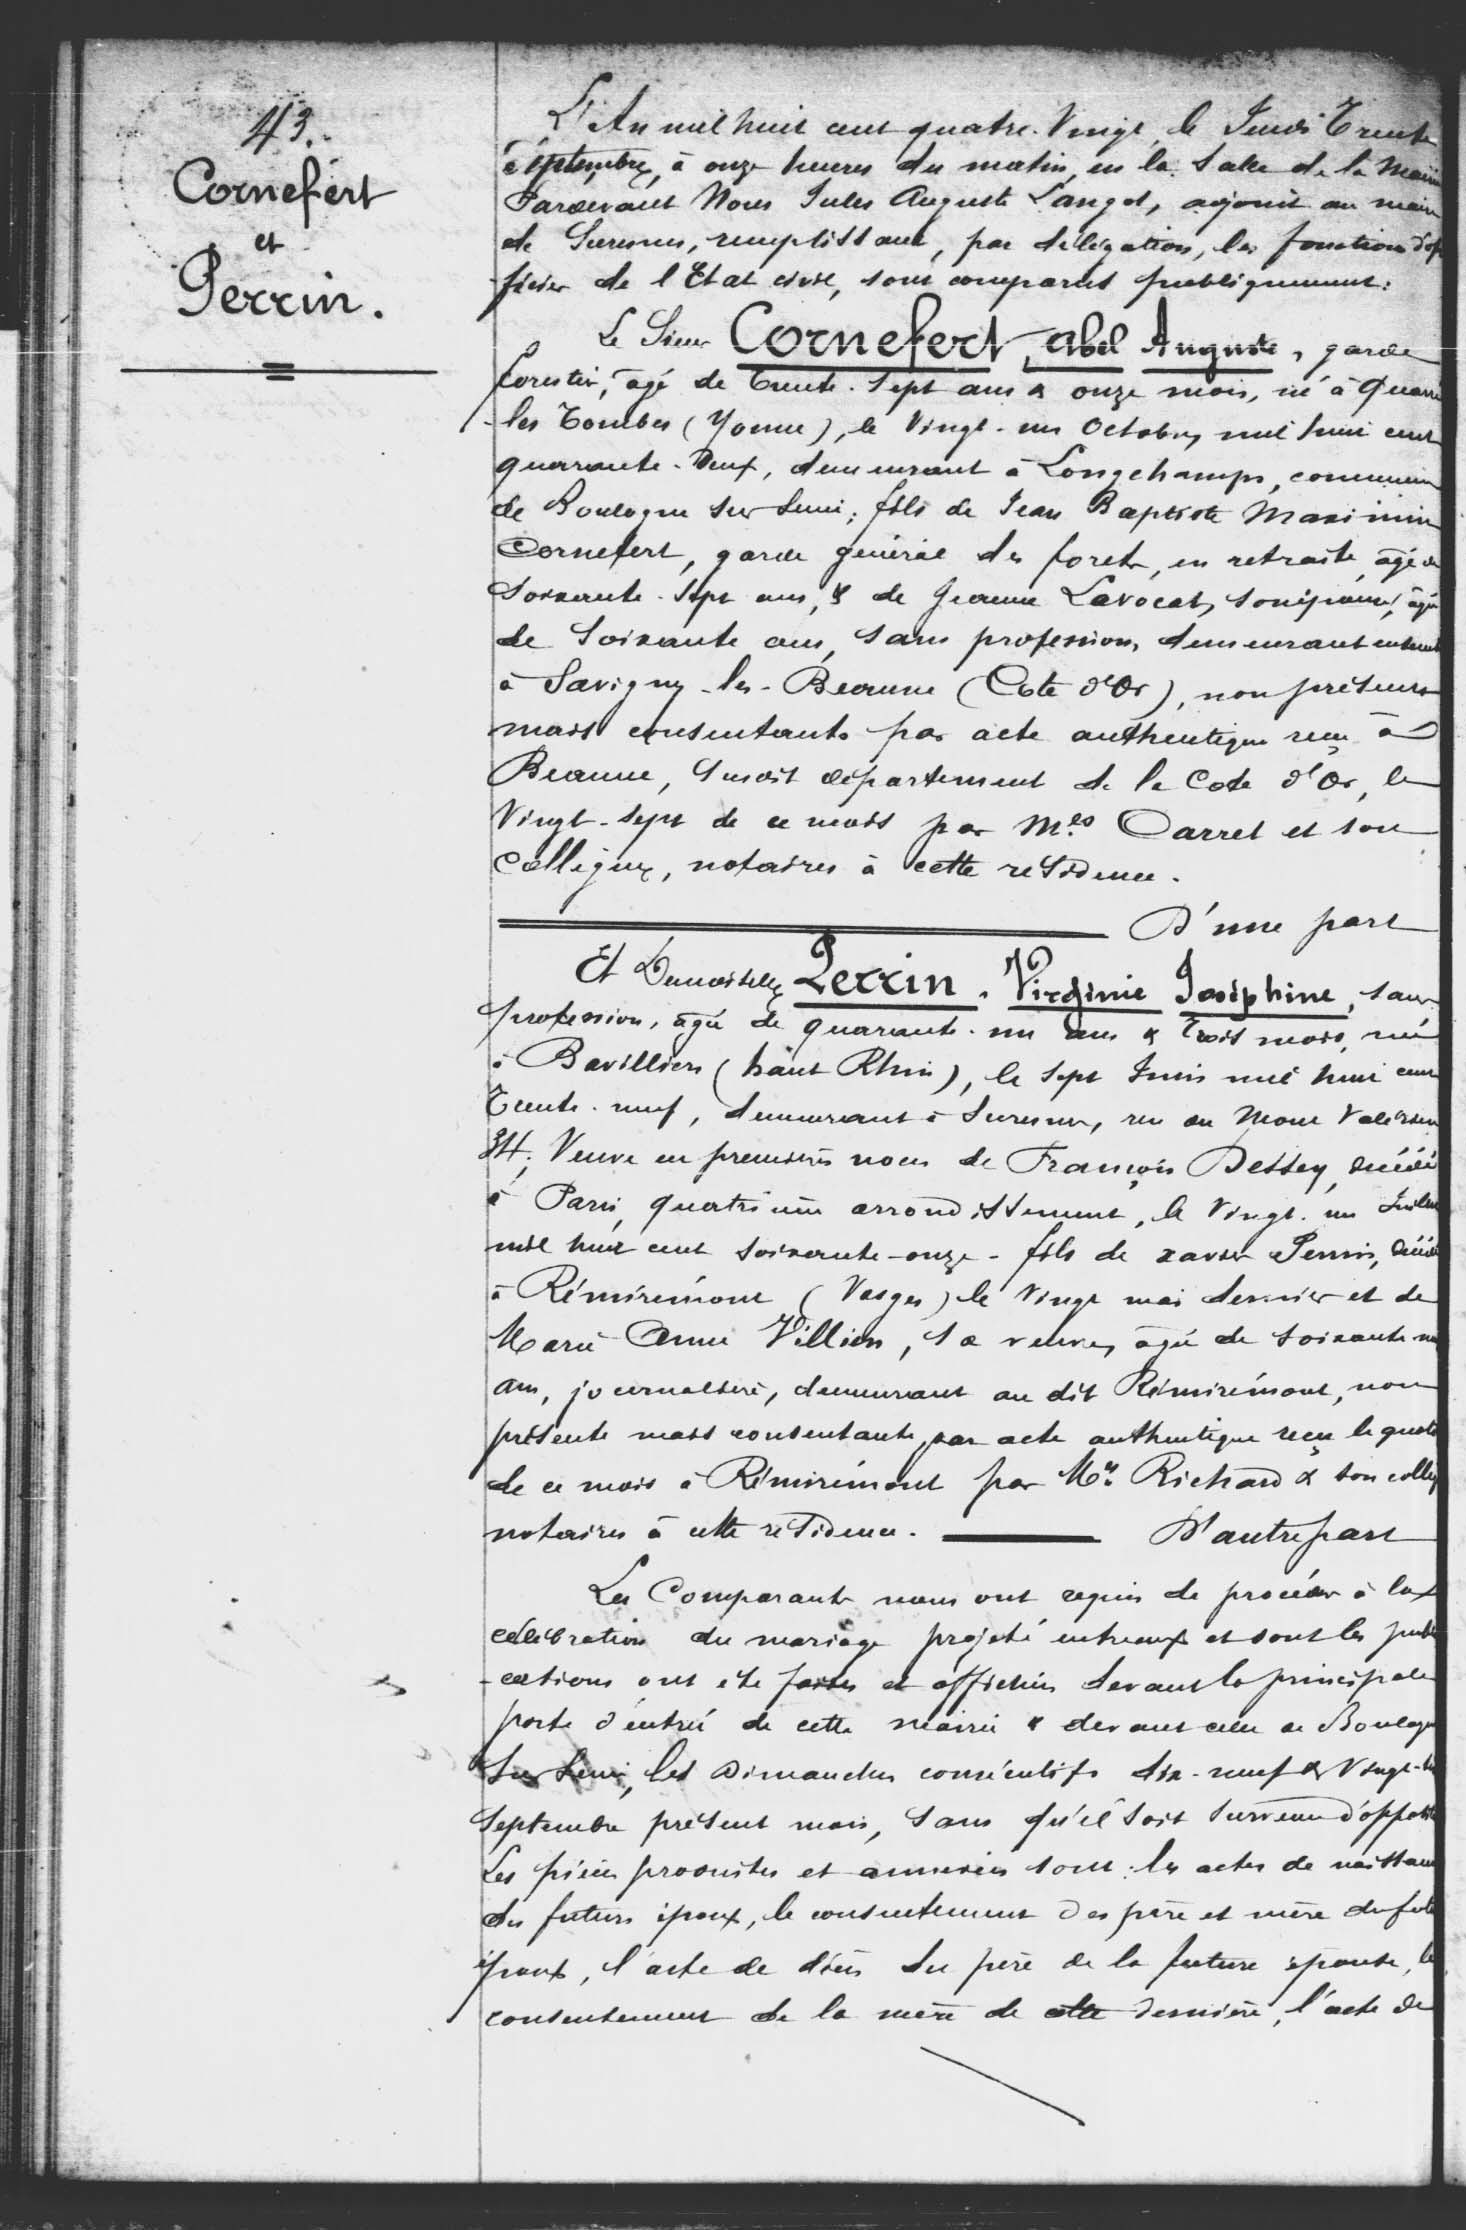43
Cornefert
et
Perrin.
L'An mil huit cent quatre Vingt le Jeudi Trente
septembre, à onze heures du matin, en la Salle de la Mairie
Pardevant Nous Jules Auguste Langot, adjoint au maire
de Turesnes, remplissant, par délégation, la fonctions d'of
ficier de l'Etat civil, sont comparus publiquement:
Le Sieur Cornefert, Abel Auguste, garde
forestier, agé de Trente sept ans & onze mois, née à Quani
les Tourbes (Yonne), le Vingt-un Octobre, mil huit cent
quarante-deux, demeurant à Longchamps, commune
de Boulogne sur Seine; fils de Jean Baptiste Maximin
Cornefert, garde général des forets, en retraite agé de
soixante-sept ans, de Jeanne Lavocat, son épouse, âgé
de Soixante ans, sans profession, demeurant ensemble
à Savigny-les-Beaunes (Cote-d'Or), non présents
mais consentants par acte authentique reçu à
Beaune, susdit département de la Cote d'Or, le
Vingt-sept de ce mois par Mes Carret et son
Collègue, notaires à cette résidence.
-D'une part
Et Damoiselle Perrin Virginie Joséphine, sans
profession, agé de quarante un ans & trois mois née
à Bavilliers (haut Rhin), le sept Juin mil huit cent
Trente-neuf, demeurant à Suresnes, rue du Mont Valérien
34; Veuve en premières noces de François Dessey, décédé
à Paris, quatrième arrondissement, le vingt-un Juillet
mil neuf cent soixante-onze-fils de Xavier Perrin, décédé
à Rémiremont (Vosges) le Vingt mai dernier et de
Marie Anne Villion, sa veuve, agé de soixante un
ans, journalière, demeurant au dit Rémiremont, non
présents mais consentants par acte authentique reçu le quatre
de ce mois à Rémiremont par Me Richard & son colleg
notaires à cette résidence. -D'autre part
Les Comparant nous ont requis de procéder à la
célébration du mariage projeté entre eux et dont les publi
-cations ont été faites et effectuées devant la principale
porte d'entrée de cette mairie & devant celles de Boulogne
sur Seine, les Dimanches consécutifs, dix-neuf & vingt-six
Septembre présent mais, sans qu'il soit survenu d'opposition
Les pièces produites et annexées sont les actes de naissance
du futurs époux, le consentement des père et mère du futur
époux, l'acte de décès du père de la future épouse, le
consentement de la mère de cette dernière, l'acte de
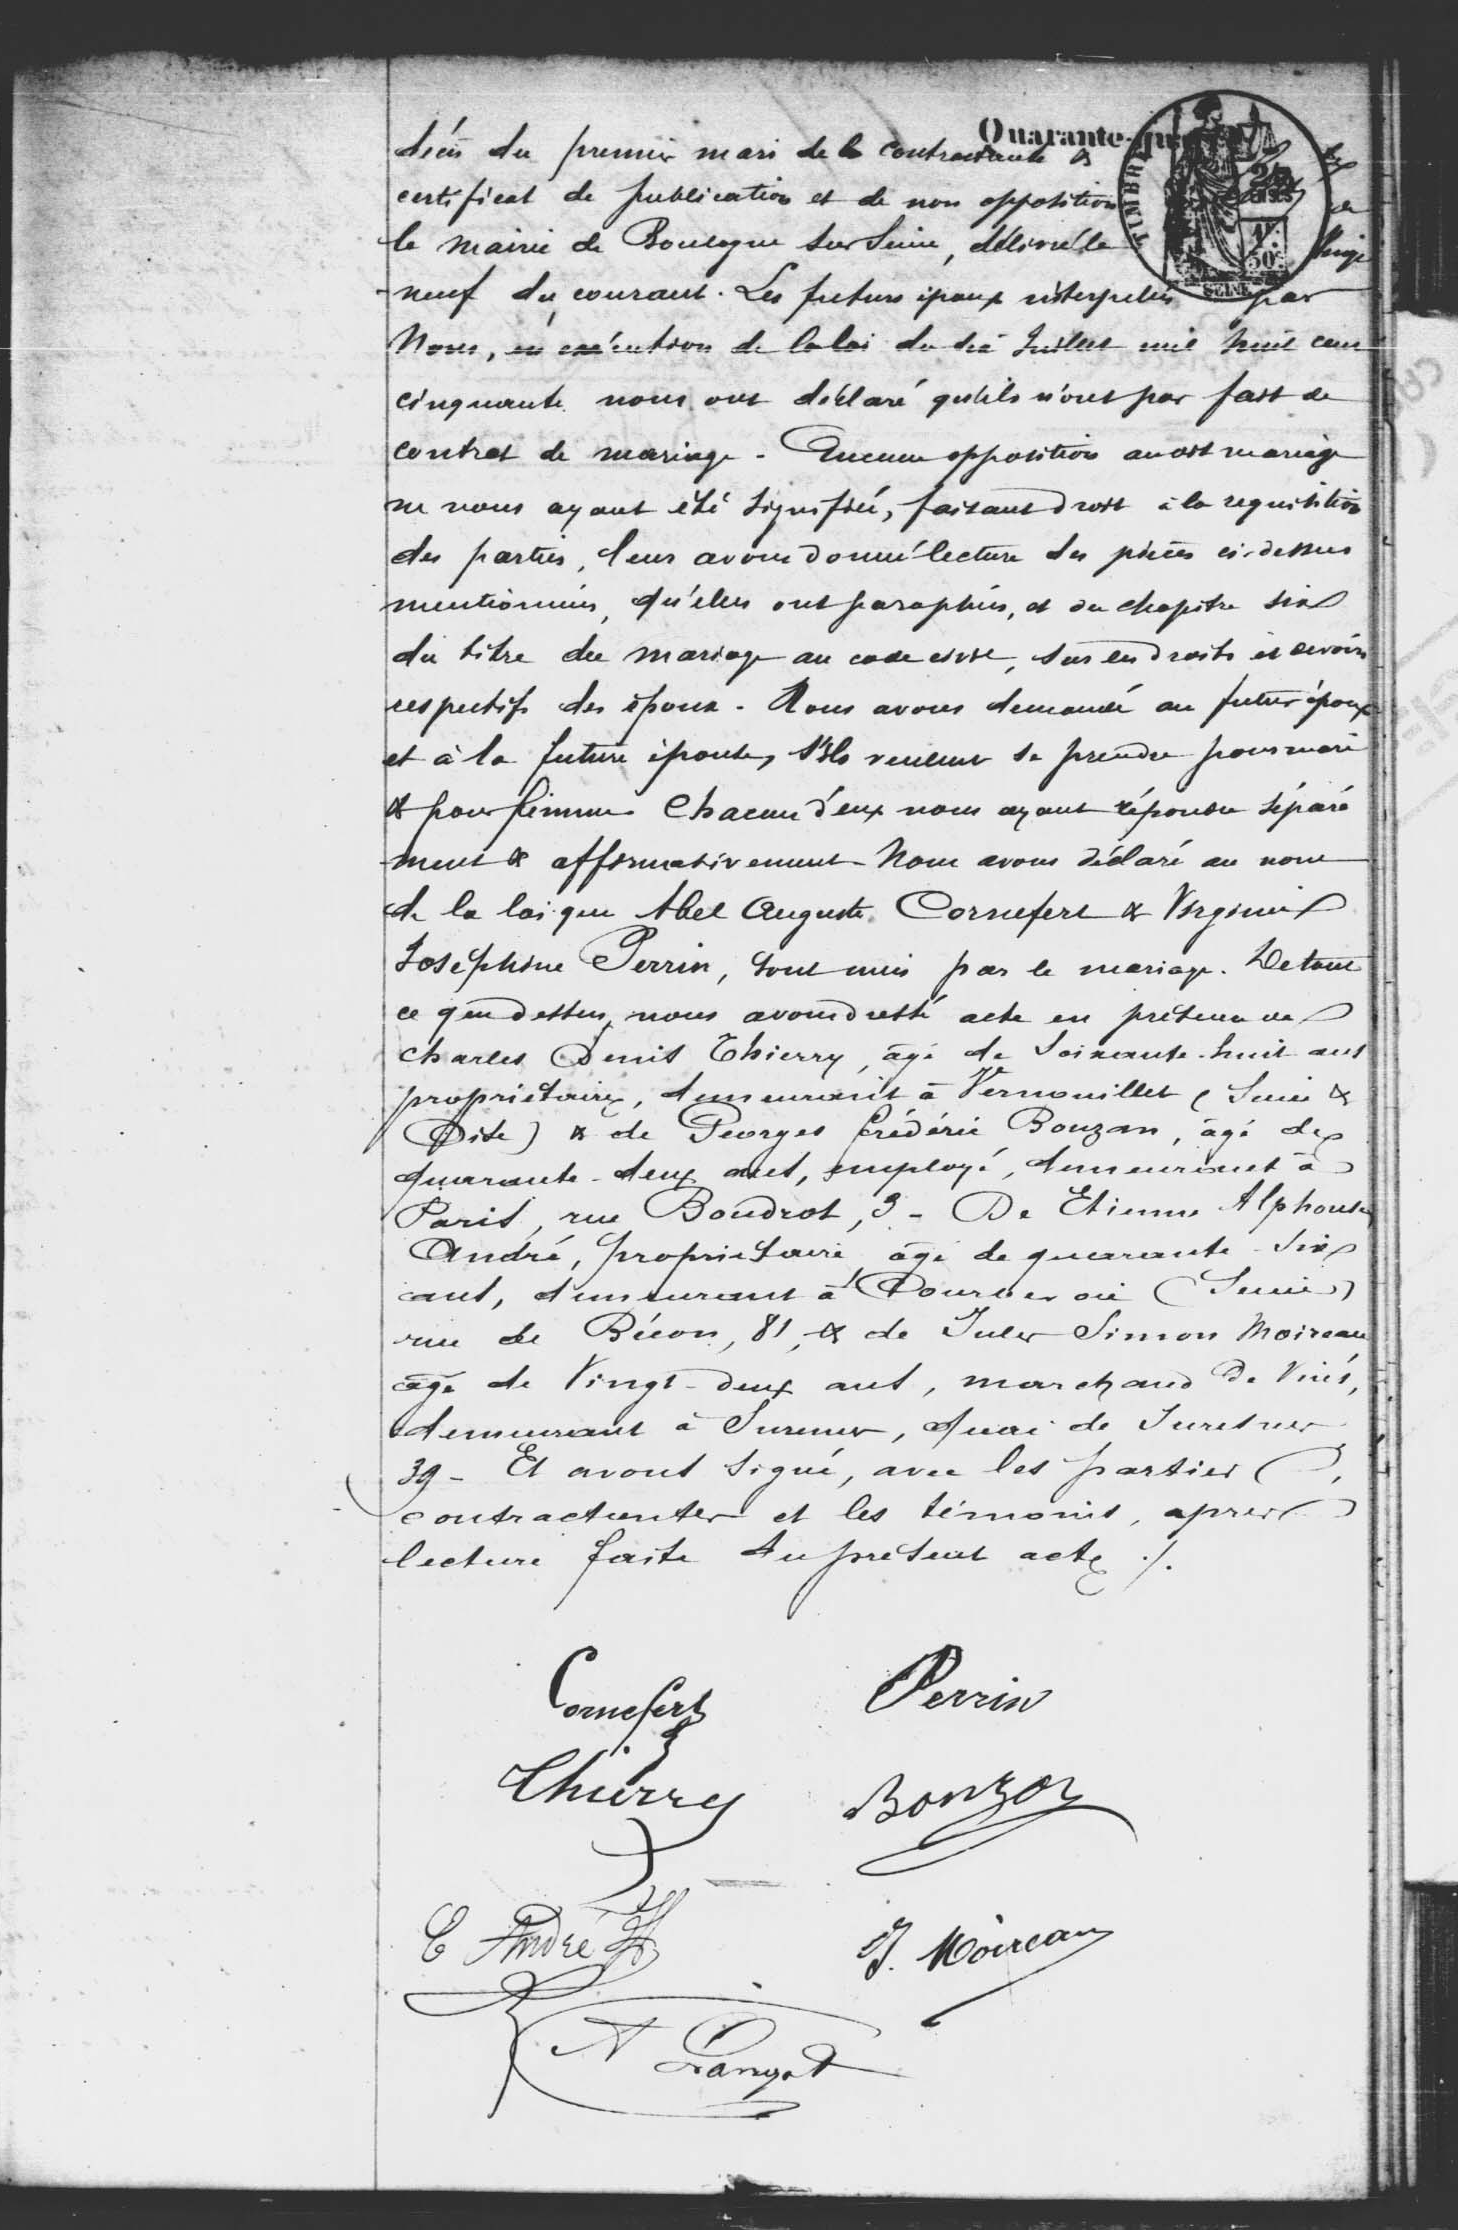décès du premier mari de la contractante & le
certificat de publication et de non opposition de
la mairie de Boulogne sur Seine, délivré le vingt
neuf du courant. Les futurs époux interpellés par
Nous,en exécution de la loi du dix Juillet mil neuf cent
cinquante nous ont déclaré qu'il n'ont pas fait de
contrat de mariage. Aucune opposition au dit mariage
ne nous ayant été signifié, faisant droit à la réquisition
des parties, leur avons donné lecture des pièces ci dessus
mentionnés, qu'elles ont paraphés, et du chapitre six
du titre du mariage au code civil, sus en droits et devoirs
respectifs des époux. Nous avons demandé au futur époux
et à la future épouse, s'ils veulent se prendre pour mari
& pour femme. Chacun d'eux nous ayant répondu séparé
-ment & affirmativement-Nous avons déclaré au nom
de la loi que Abel Auguste Cornefert & Virginie
Joséphine Perrin, sont unis par le mariage. De tout
ce que dessus nous avons dressé acte en présence de
Charles Denis Thierry, agé de Soixante-huit-ans
propriétaires, demeurant à Vernouillet (Seine &
Oise) & de Georges Frédéric Bouzan, agé de
quarante-deux ans, employé, demeurant à
Paris, rue Boudrot, 3-De Etienne Alphonse
André, propriétaire âgé de quarante-six
ans, demeurant à Courbevoie (Seine)
rue de Bécon, 81, & de Jules Simon Moireau
agé de Vingt-deux ans, marchand de Vins,
demeurant à Suresnes, quai de Suresnes
39-Et avons signé, avec les parties
contractants et les témoins, après
lecture fait du présent acte ./.